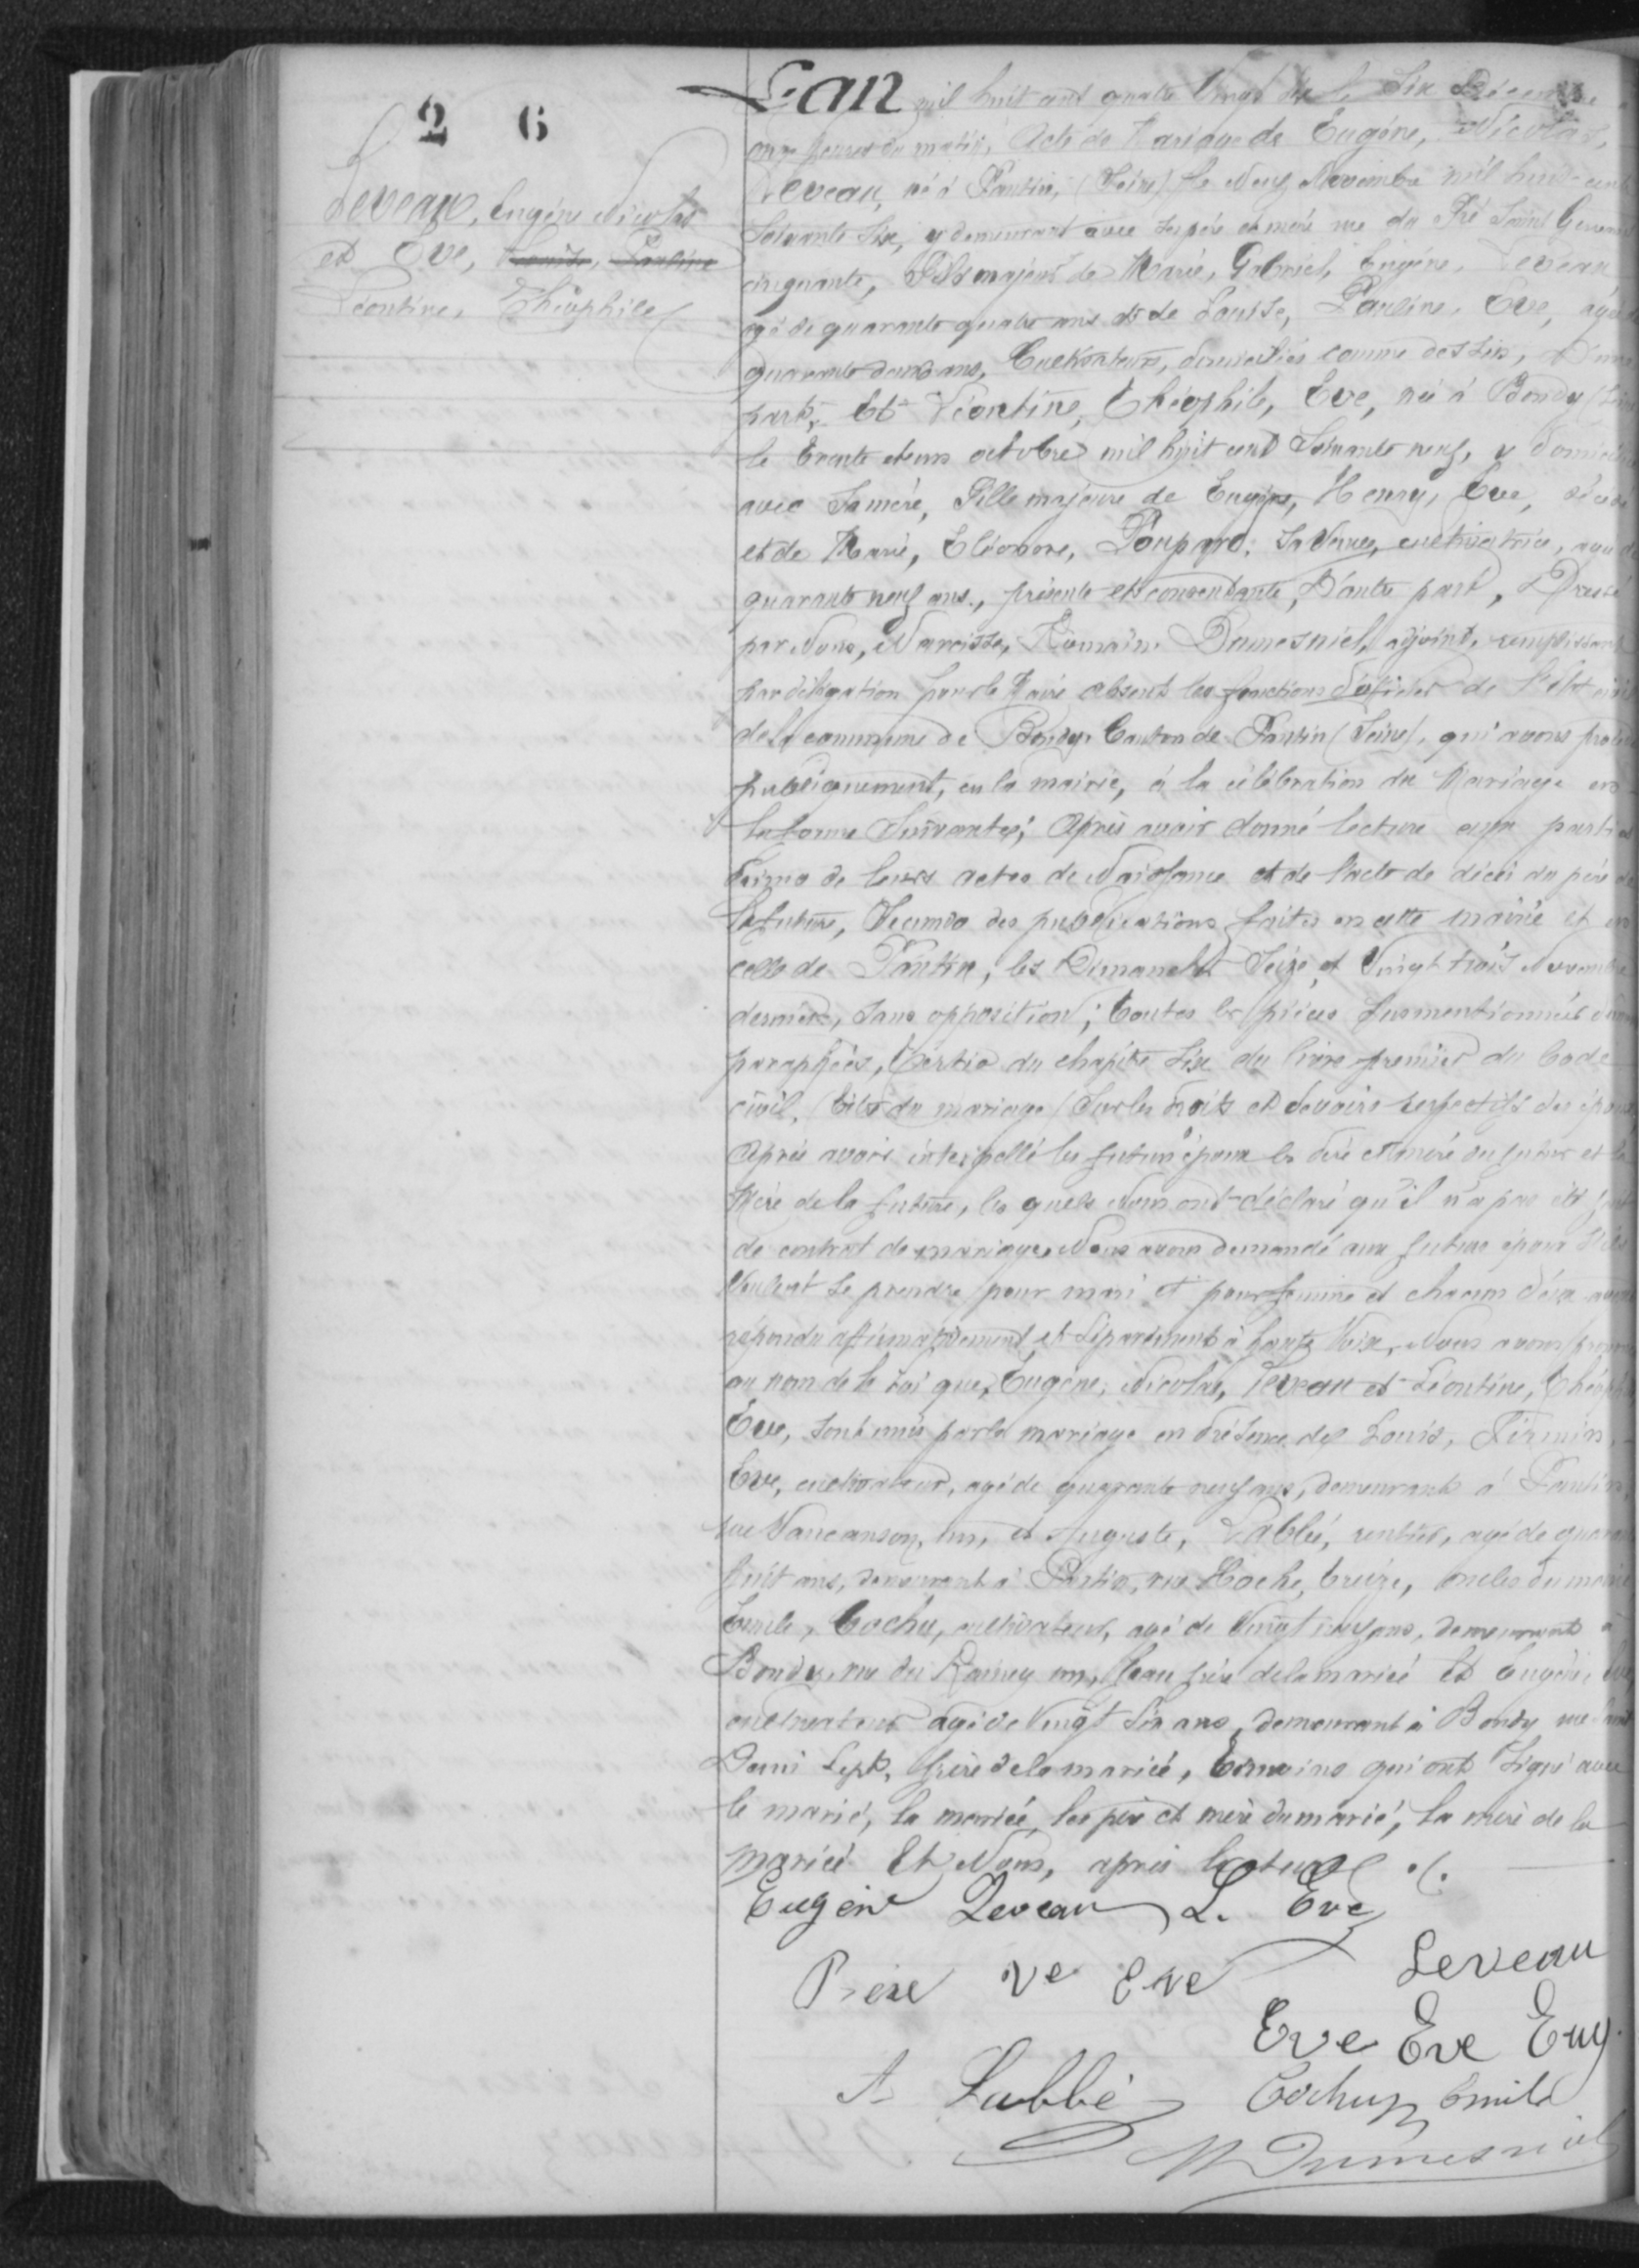26
Leveau, Eugène Nicolas
et Eve, Louise, Pauline
Léontine, Théophile
L'an mil huit cent quatre vingt dix, six Décembre
onze heures du matin, Acte de mariage de Eugène, Nicolas,
Neveau, né à Pantin, (Seine) Le seize Novembre mil huit cent
Soixante Six, y demeurant avec ses père et mère rue du Pré Saint Germain
cinquante, fils majeur de Marie, Gabriel, Eugène, Leveau,
âgé de quarante quatre ans et de Louise, Pauline, Eve, âgée
quarante deux ans, Cultivateurs, domicilliés comme dessus, D'une
part, Et Léontine Théophile, Eve, née à Bondy (Seine)
le trente et un octobre mil huit cent Soixante neuf, y domicilié
avec sa mère, Fille majeure de Eugène, Henry, Eve, décédé
et de René, Eleonore, Ponpard: sa veuve, cultivatrice, âgée de
quarante neuf ans, présente et consentante, D'autre part, Dressé
par Nous, et Narcisse, Romain Demesne, adjoint, remplissant
par délégation pour le Maire absent les fonctions d'officier de l'Etat civil
de la commune de Bondy Canton de Pantin (Seine), qui avons procédé
publiquement, en la mairie, à la célébration du marriage en
la forme suivante; Après avoir donné lecture aux parties
Primo de leurs actes de naissance et de l'acte de décès du père de
la future, Secundo des publications faites en cette mairie et en
celle de Pantin, les Dimanches Seize et Vingt trois Novembre
dernier, sans opposition; toutes les pièces susmentionnées dûment
paraphées, partie du chapitre six du livre premier du Code
civil, titre du mariage, sur les droits et devoirs respectifs des époux
après avoir interpellé les futurs époux les père et mère du futur et la
mère de la future, lesquels nous ont déclaré qu'il n'a pas été fait
de contrat de mariage Nous avons demandé aux futurs époux s'ils
veulent se prendre pour mari et pour femme et chacun d'eux ayant
répondu affirmativement et séparément à haute voix, Nous avons prononcé
au nom de la Loi que, Eugène, Nicolas, Leveau et Léontine, Théophile
Eve, sont unis par le mariage en présence de Louis, Firmin,
Eve, cultivateur, agé de quarante neuf ans, demeurant à Pantin
rue Vaucanson, un et Auguste, Lablée, rentier, agé de quarante
huit ans, demeurant à Pantin, rue Hoche, Treize, oncles du marié
Emile, Cocher, cultivateur, agé de vingt cinq ans, demeurant à
Bondy, rue du Raincy un beau frère de la mariée Et Eugène Eve
cultivateur agé de vingt six ans, demeurant à Bondy, rue Saint
Denis Sept, frère de la mariée, témoins qui ont signée avec
le marié, la mariée, les père et mère du mariée, la mère de la
mariée Et Nous, après lecture./.
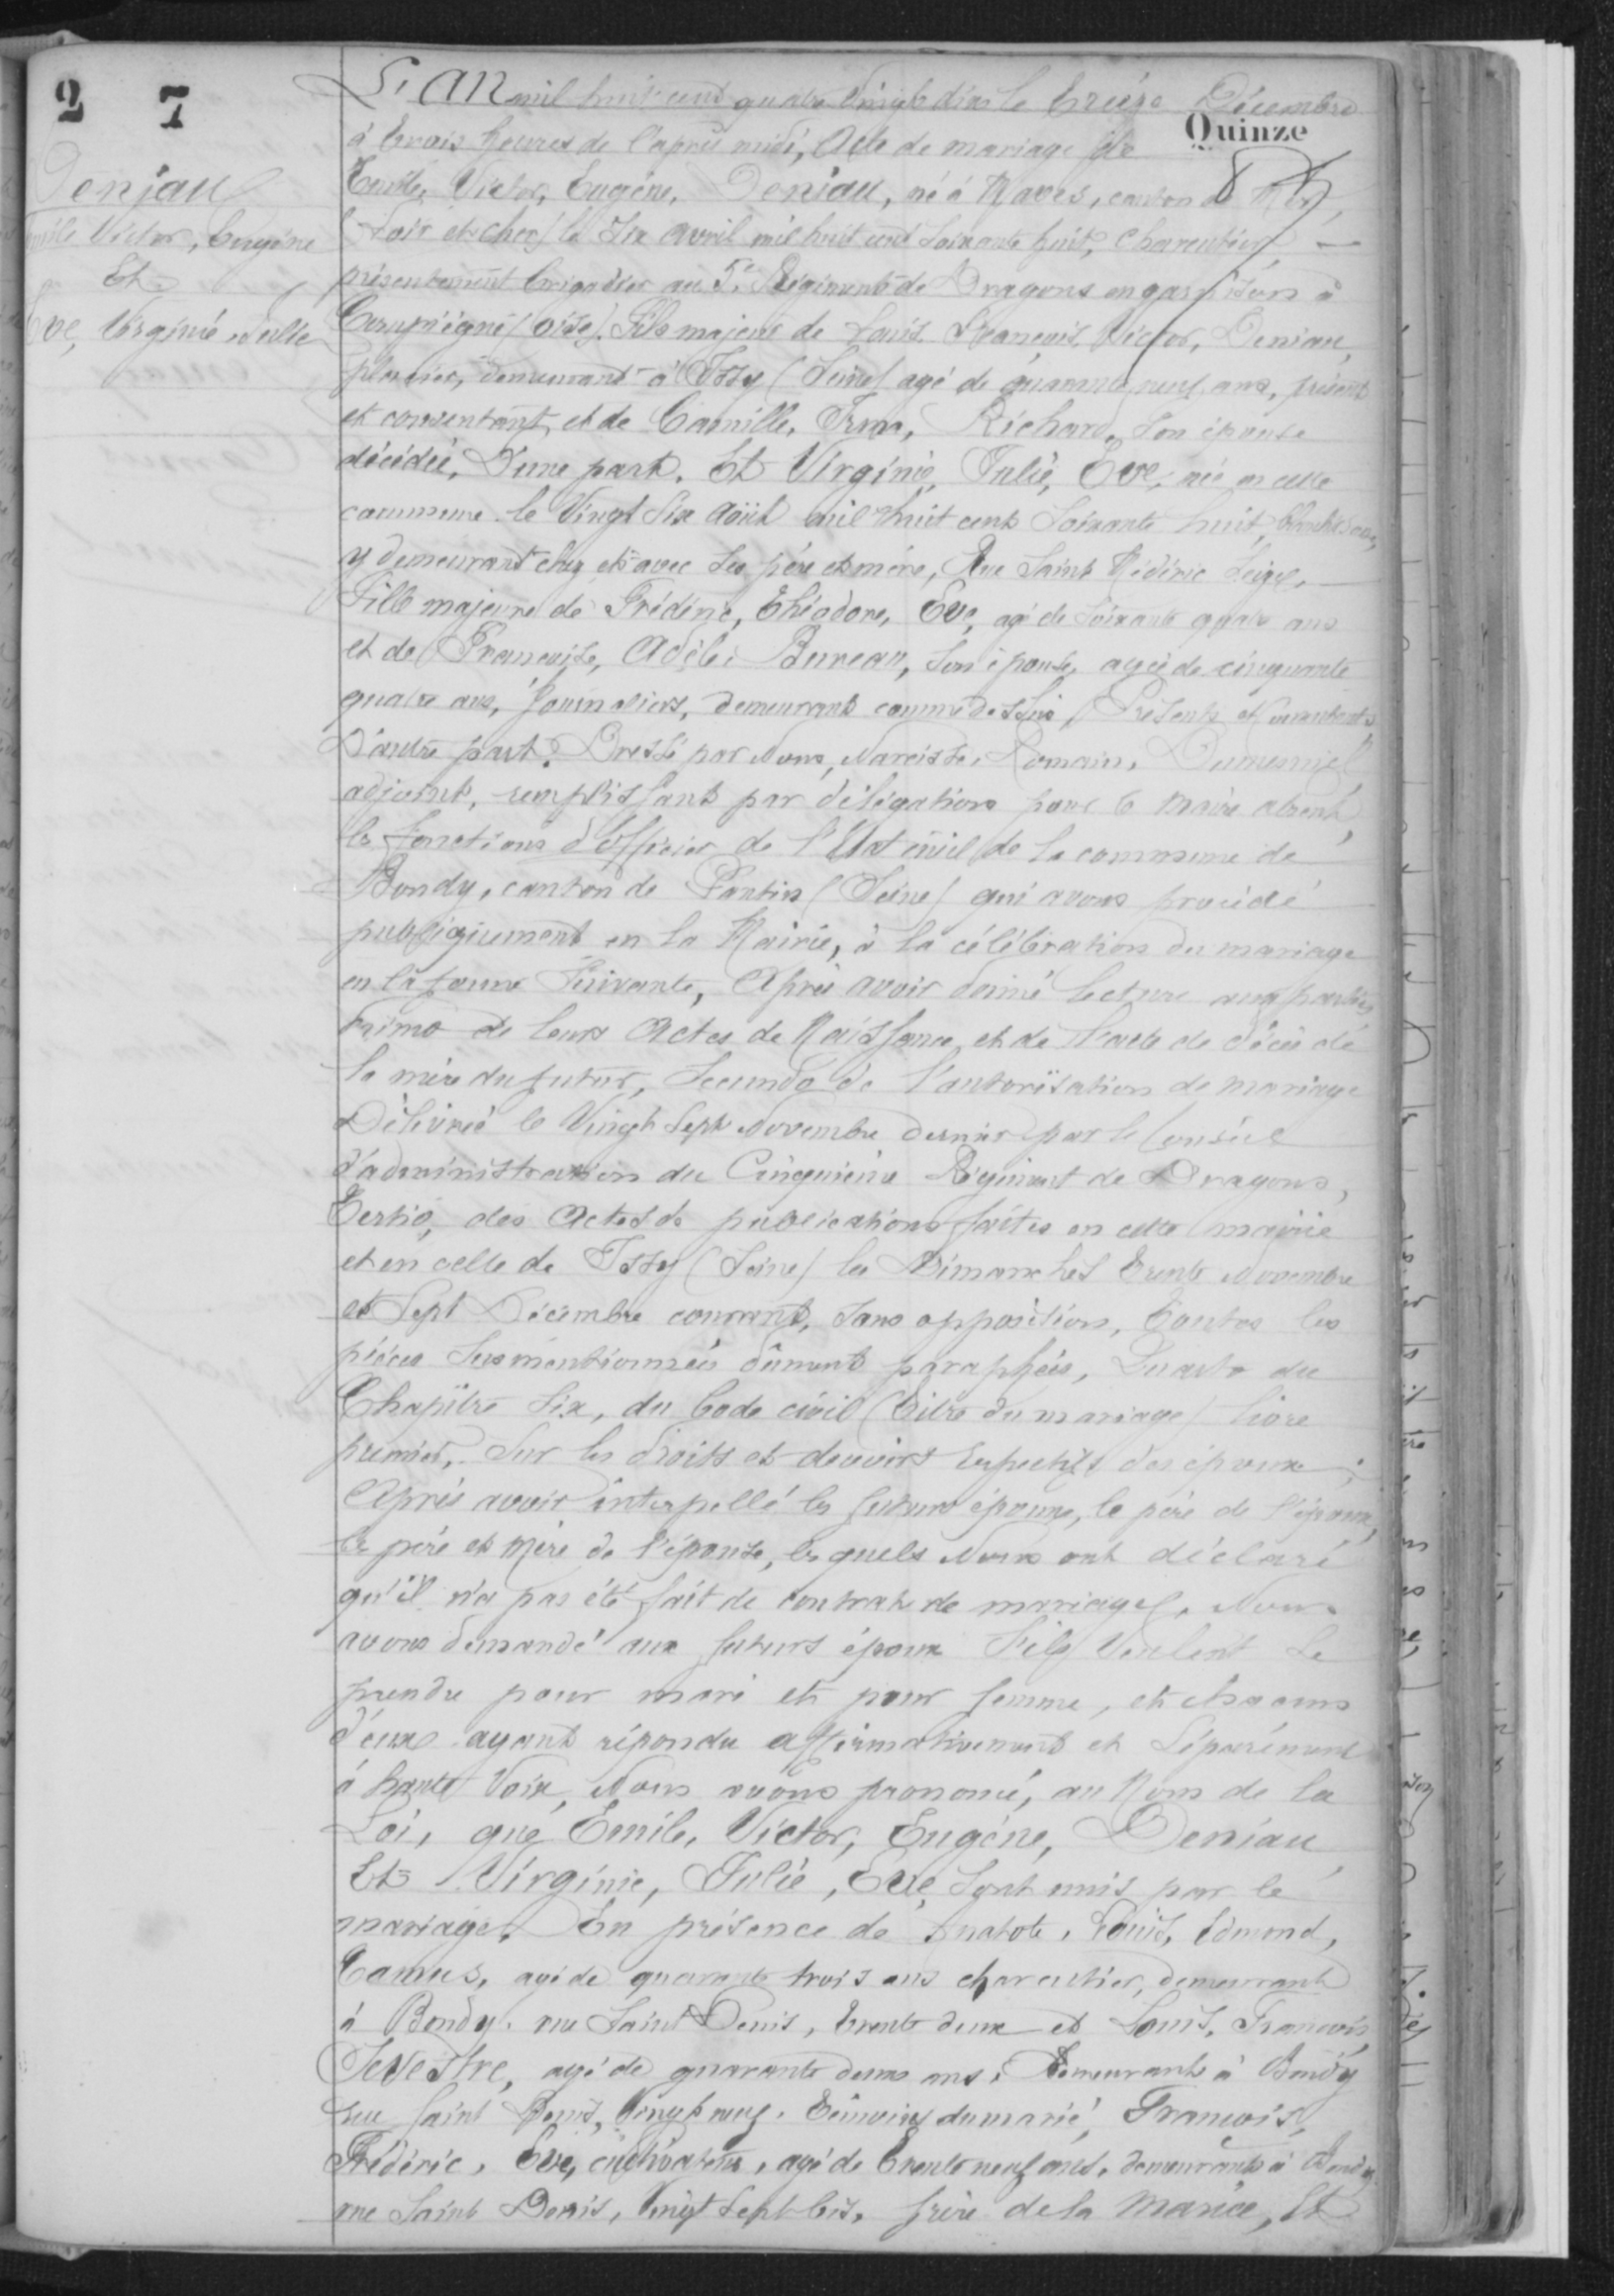27
Penjan
Emile Victor Eugène
Et
Eve, Eugène Julie
L'an mil huit cent quatre vingt dix le treize Décembre
à trois heures de l'après midi, Acte de mariage de
Emile Victor Eugène Deniau, né à Naves, canton de Mer
(Loir et Cher) le six avril mil huit cent Soixante huit, charentier
présentement brigadier du 5e Régiment de Dragons en garnison à
Compiègne (Oise) Fils majeur de Louis François Victor, Deniau,
plombier demeurant à Issy (seine) âgé de quarante neuf ans, présent
et consentant, et de Camille, Irma, Richard, son épouse
décédée, D'une part, Et Virginie, Julie, Eve, née en cette
commune le vingt six aoüt mil huit cent soixante huit, blanchisseuse,
y demeurant et avec ses père et mère, Rue Saint Frédéric Seize,
Fille majeure de Frédine, Théodore, Eve, âgé de soixante quatre ans
et de Femeuise, Adèle, Buneau, son épouse, agée de cinquante
quatre ans, journaliers, demeurant comme dessus, Présents et consentants
D'autre part. Dressé par nous, Narcisse, Romain, Dumesniel,
adjoint, remplissant par délégation pour le Maire absent,
les fonctions d'officier de l'Etat civil de La commune de
Bondy, canton de Pantin (Seine) qui avons procédé
publiquement en la Mairie, à la célébration du mariage
en la forme suivante, Après avoir donné lecture aux parties
primo de leurs Actes de Naissance, et de l'acte de décès de
la mère du futur, Secundo de l'autorisation de mariage
Délivré le Vingt Sept Novembre dernier par le Conseil
d'administration du Cinquième Régiment de dragons,
Tertio, des Actes de publications faites en cette mairie
et en celle de Issy (Seine) les Dimanches trente novembre
et Sept Décembre courant, sans opposition, toutes les
pièces susmentionnées dûment paraphées, Les actes du
Chapitre Six, du Code civil (Titre du mariage) Livre
premier sur les droits et devoirs respectifs des époux
Après avoir interpellé les futurs époux, le père de l'épouse,
le père et mère de l'épouse, lesquels Nous ont déclaré
qu'il n'a pas été fait de contrat de mariage, Nous
avons demandé aux futurs époux s'ils veulent se
prendre pour mari et pour femme, et chacuns
d'eux ayant répondu affirmativement et séparément
à haute voix, Nous avons prononcé, au nom de la
Loi, que Emile, Victor, Eugène, Deniau,
Et Virginie, Julie, Eve, sont unis par le
mariage. En présence de Anatole, Louis, Edmond,
Camus, agé de quarante trois ans charretier, demeurant
à Bondy, rue Saint Denis, trente deux et Louis, François
Senestre, agé de quarante deux ans et demeurant à Bondy
rue Saint Denis, vingt neuf témoins du marié François
Frédéric, Eve, cultivateur, agé de trente neuf ans, demeurant à Bondy,
rue Saint Denis, vingt sept bis, frère de la mariée, Et